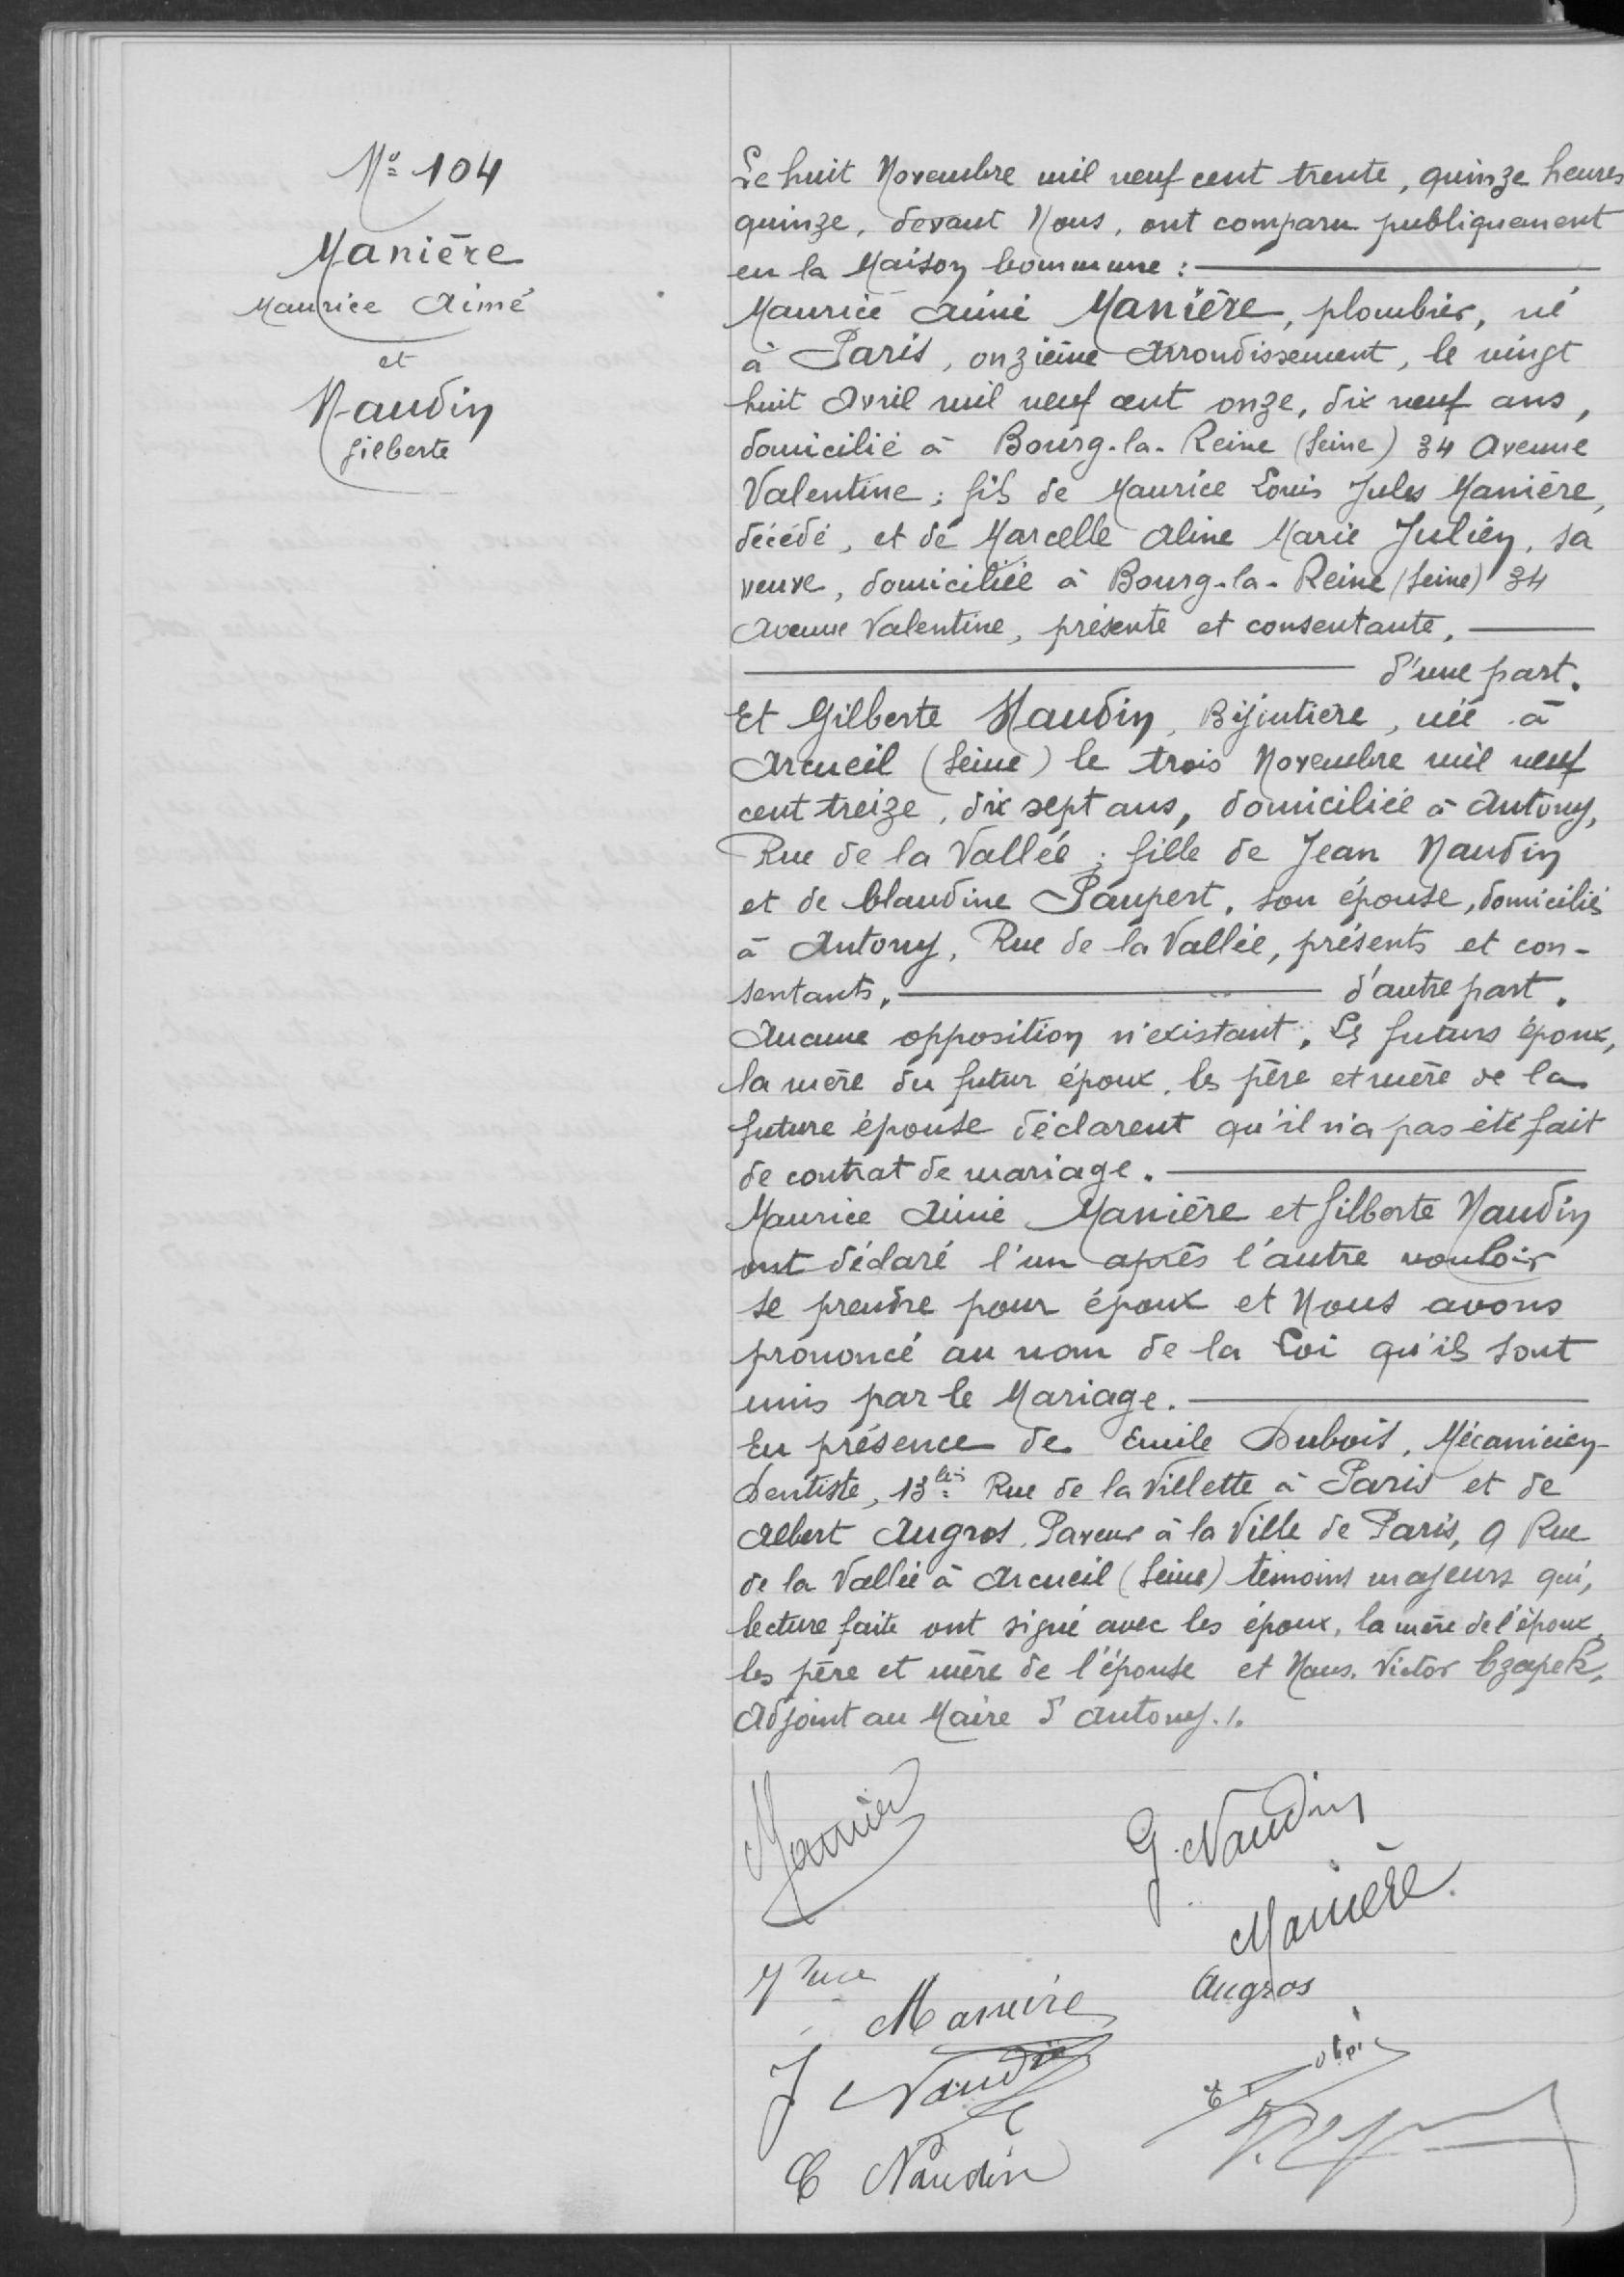N°104
Manière
Maurice Aimé
et
Naudin
Gilberte
Le huit Novembre mil neuf cent trente, quinze heures
quinze, devant Nous, ont comparu publiquement
en la Maison commune: -
Maurice Aimé Manière, plombier, né
à Paris, onzième arrondissement, le vingt
huit avril mil neuf cent onze, dix neuf ans,
domicilié à Bourg-la-Reine (Seine) 34 Avenue
Valentine: fils de Maurice Louis Jules Manière,
décédé, et de Marcelle Aline Marie Julien, sa
veuve, domiciliée à Bourg-la-Reine (Seine) 34
Avenue Valentine, présente et consentante, -
-d'autre part.
Et Gilberte Maudin, régulière, née à
Arcueil (Seine) le trois Novembre mil neuf
cent treize, dix sept ans, domiciliée à Autony,
Rue de la Vallée: fille de Jean Naudin
et de Blandine Paupert, bon épouse, domiciliés
à Antony, Rue de la Vallée, présents et con-
sentants. -d'autre part.
Aucune opposition n'existant. Les futurs époux,
la mère du futur époux, les père et mère de la
future épouse déclarent qu'il n'a pas été fait
de contrat de mariage.-
Maurice Aimé Manière et Gilberte Naudin
ont déclaré l'un après l'autre vouloir
se prendre pour époux et Nous avons
prononcé au nom de la Loi qu'ils sont
unis par le Mariage.
En présence de Emile Dubois, Mécanicien
dentiste, 13 bis Rue de la villette à Paris et de
Albert Augros Paveur à la Ville de Paris, 9 Rue
de la Vallée à Arcueil (Seine) témoins majeurs qui,
lecture faite ont signé avec les époux, la mère de l'époux,
les père et mère de l'épouse et Nous, Victor Egopek,
Adjoint au Maire d'Antony ./.
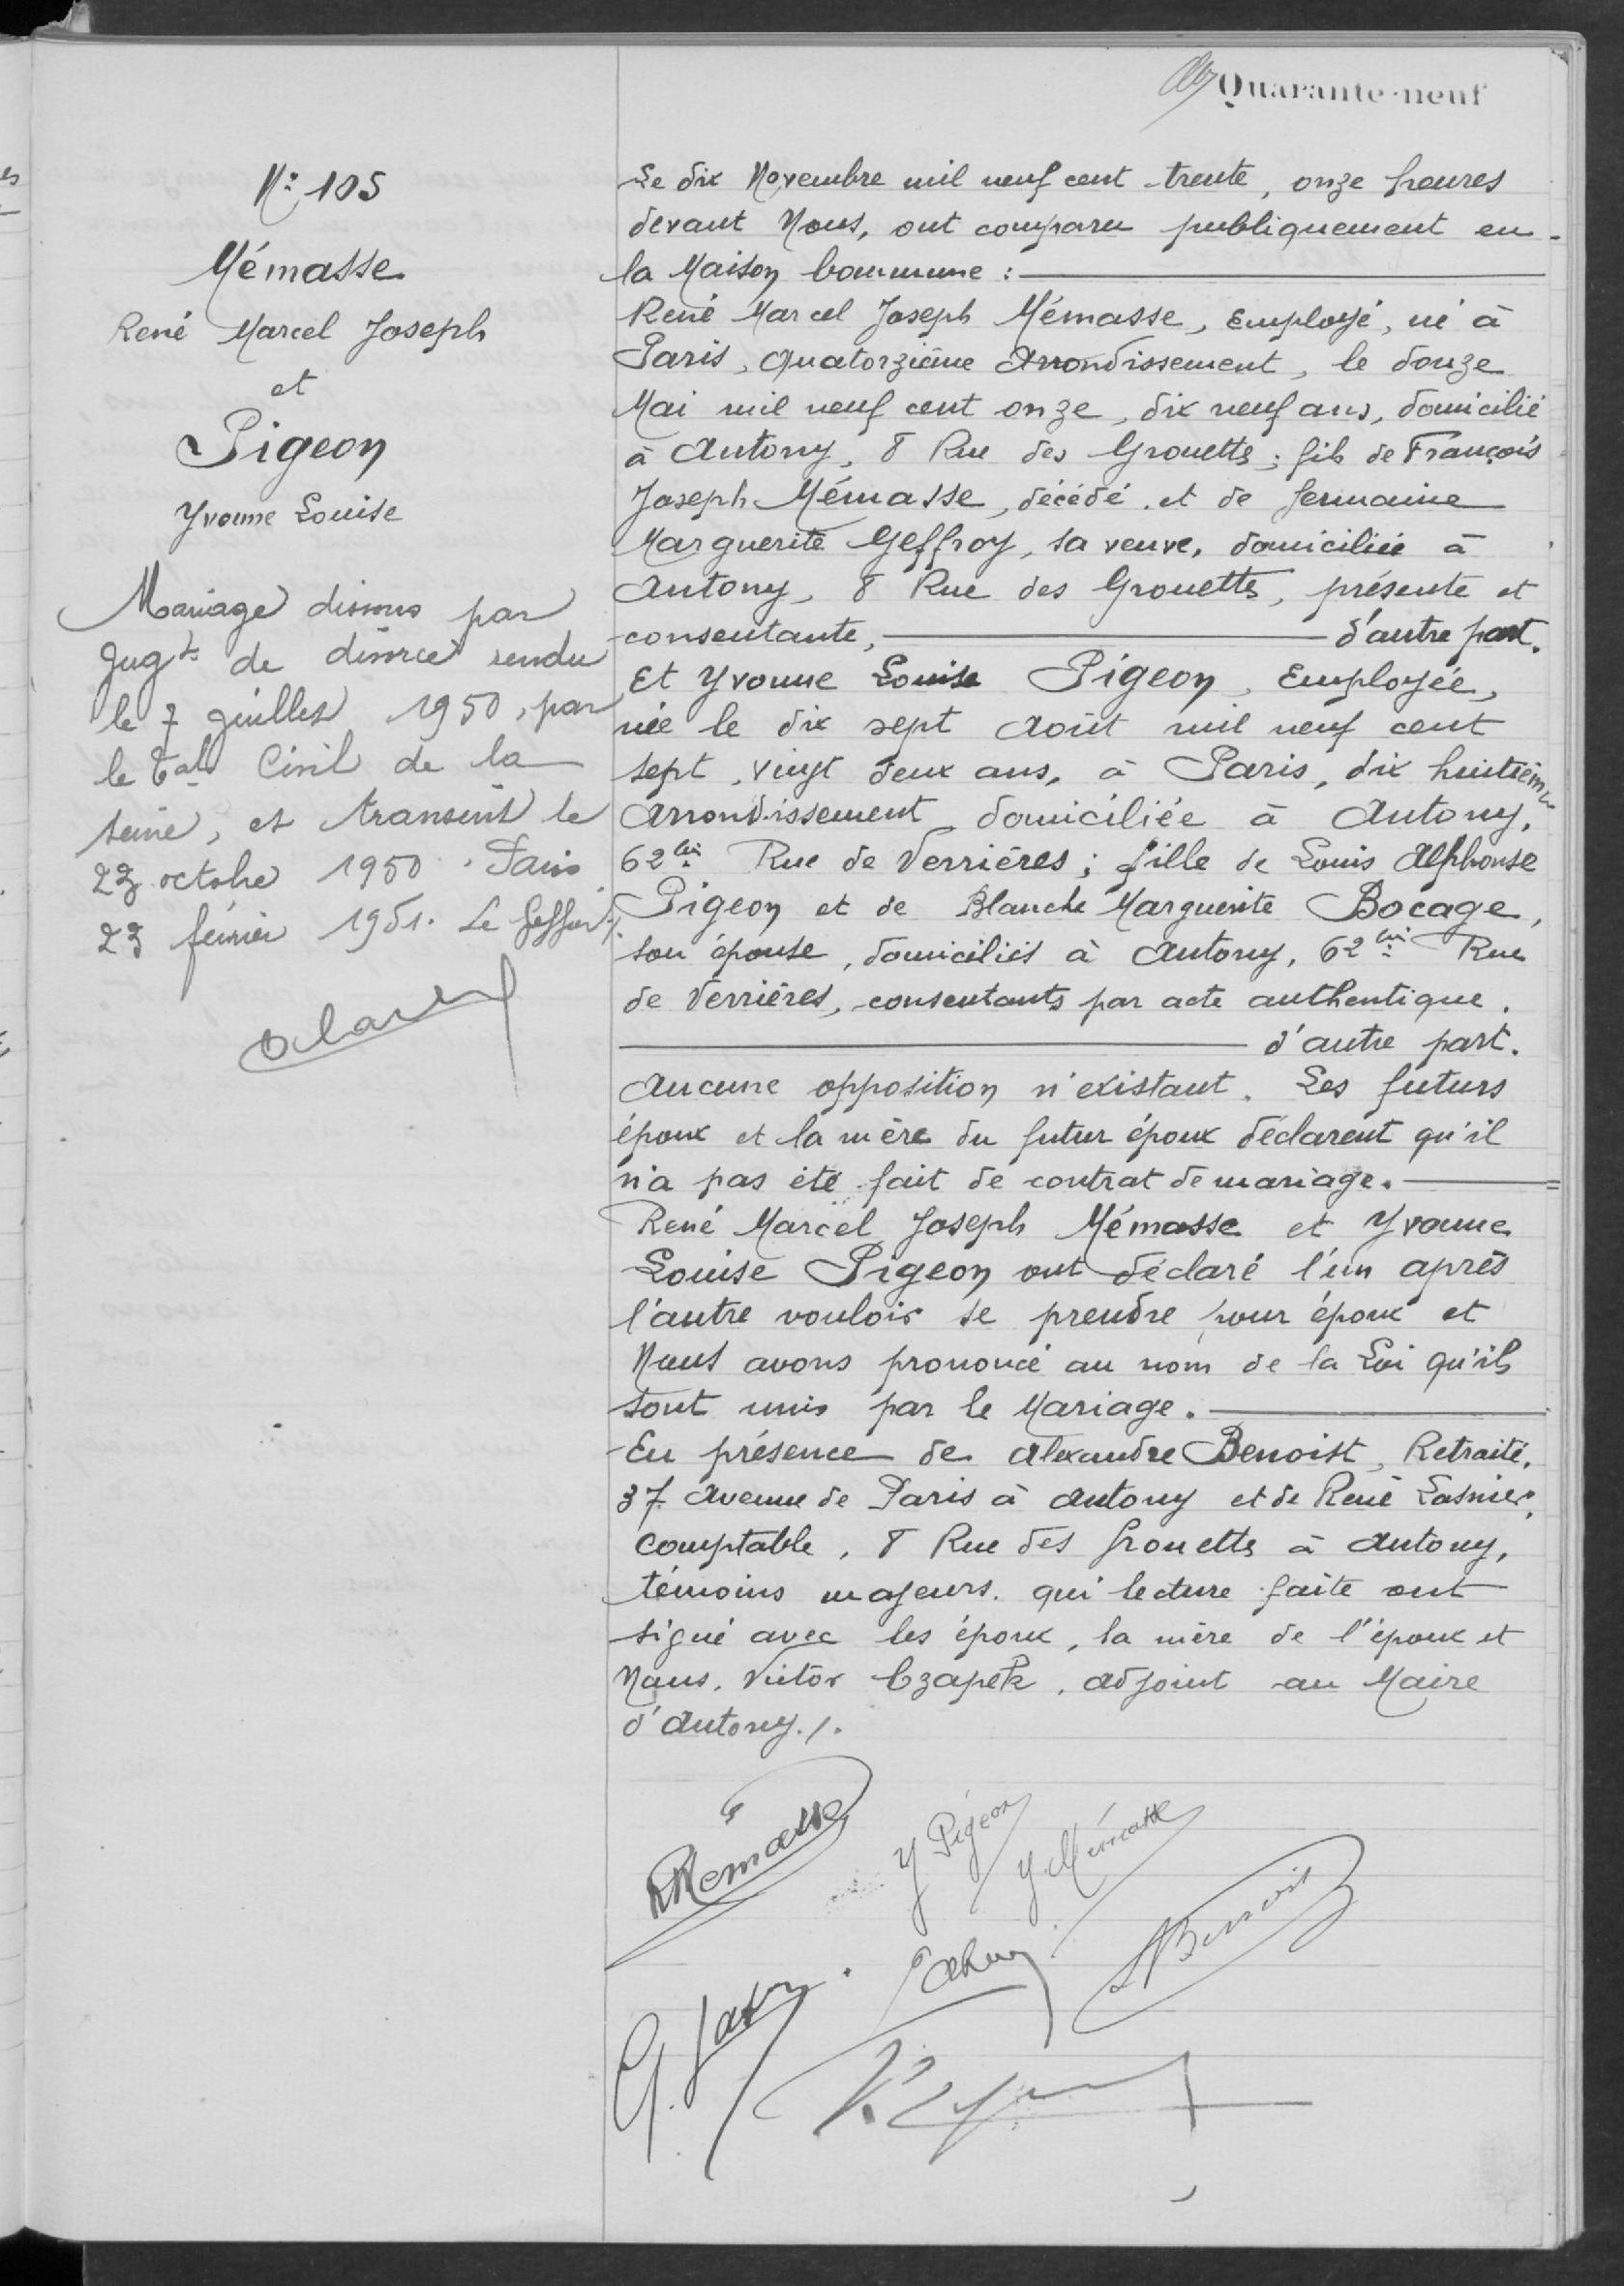N°105
Mémasse
René Marcel Joseph
et
Pigeon
Yvonne Louise
Le dix Novembre mil neuf cent-trente, onze heures
devant Nous, ont comparu publiquement en
la Maison Commune: -
René Marcel Joseph Mémasse, employé, né à
Paris, quatorzième arrondissement, le douze
Mai mil neuf cent onze, dix neuf ans, domicilié
à Antony, 8 Rue des Grouetts; fils de François
Joseph Mémasse, décédé, et de Germaine
Marguerite Geffroy, sa veuve, domiciliée à
Antony, 8 Rue des Grouetts, présente et
consentante, -d'autre part.
Et Yvonne Louise Pigeon, Employée,
née le dix sept août mil neuf cent
sept vingt deux ans, à Paris, dix huitième
arrondissement, domiciliée à Antony,
62 bis Rue de Verrières; fille de Louis Alphonse
Pigeon et de Blanche Marguerite Bocage,
son épouse, domiciliés à Antony, 62 bis Rue
de Verrières, consentants par acte authentique.
-d'autre part.
Aucune opposition n'existant. Les futurs
époux et la mère du futur époux déclarent qu'il
n'a pas été fait de contrat de mariage.-
René Marcel Joseph Mémasse et Yvonne
Louise Pigeon ont déclaré l'un après
l'autre vouloir se prendre pour époux et
Nous avons prononcé au nom de la Loi qu'ils
sont unis par le Mariage. -
En présence de Alexandre Benoist, Retraité,
37 Avenue de Paris à Antony et de René Losnier,
comptable, 8 Rue des Grouetts à Antony,
témoins majeurs qui lecture faite ont
signé avec les époux, la mère de l'époux et
nous, Victor Egapek, adjoint au Maire
d'Antony ./.